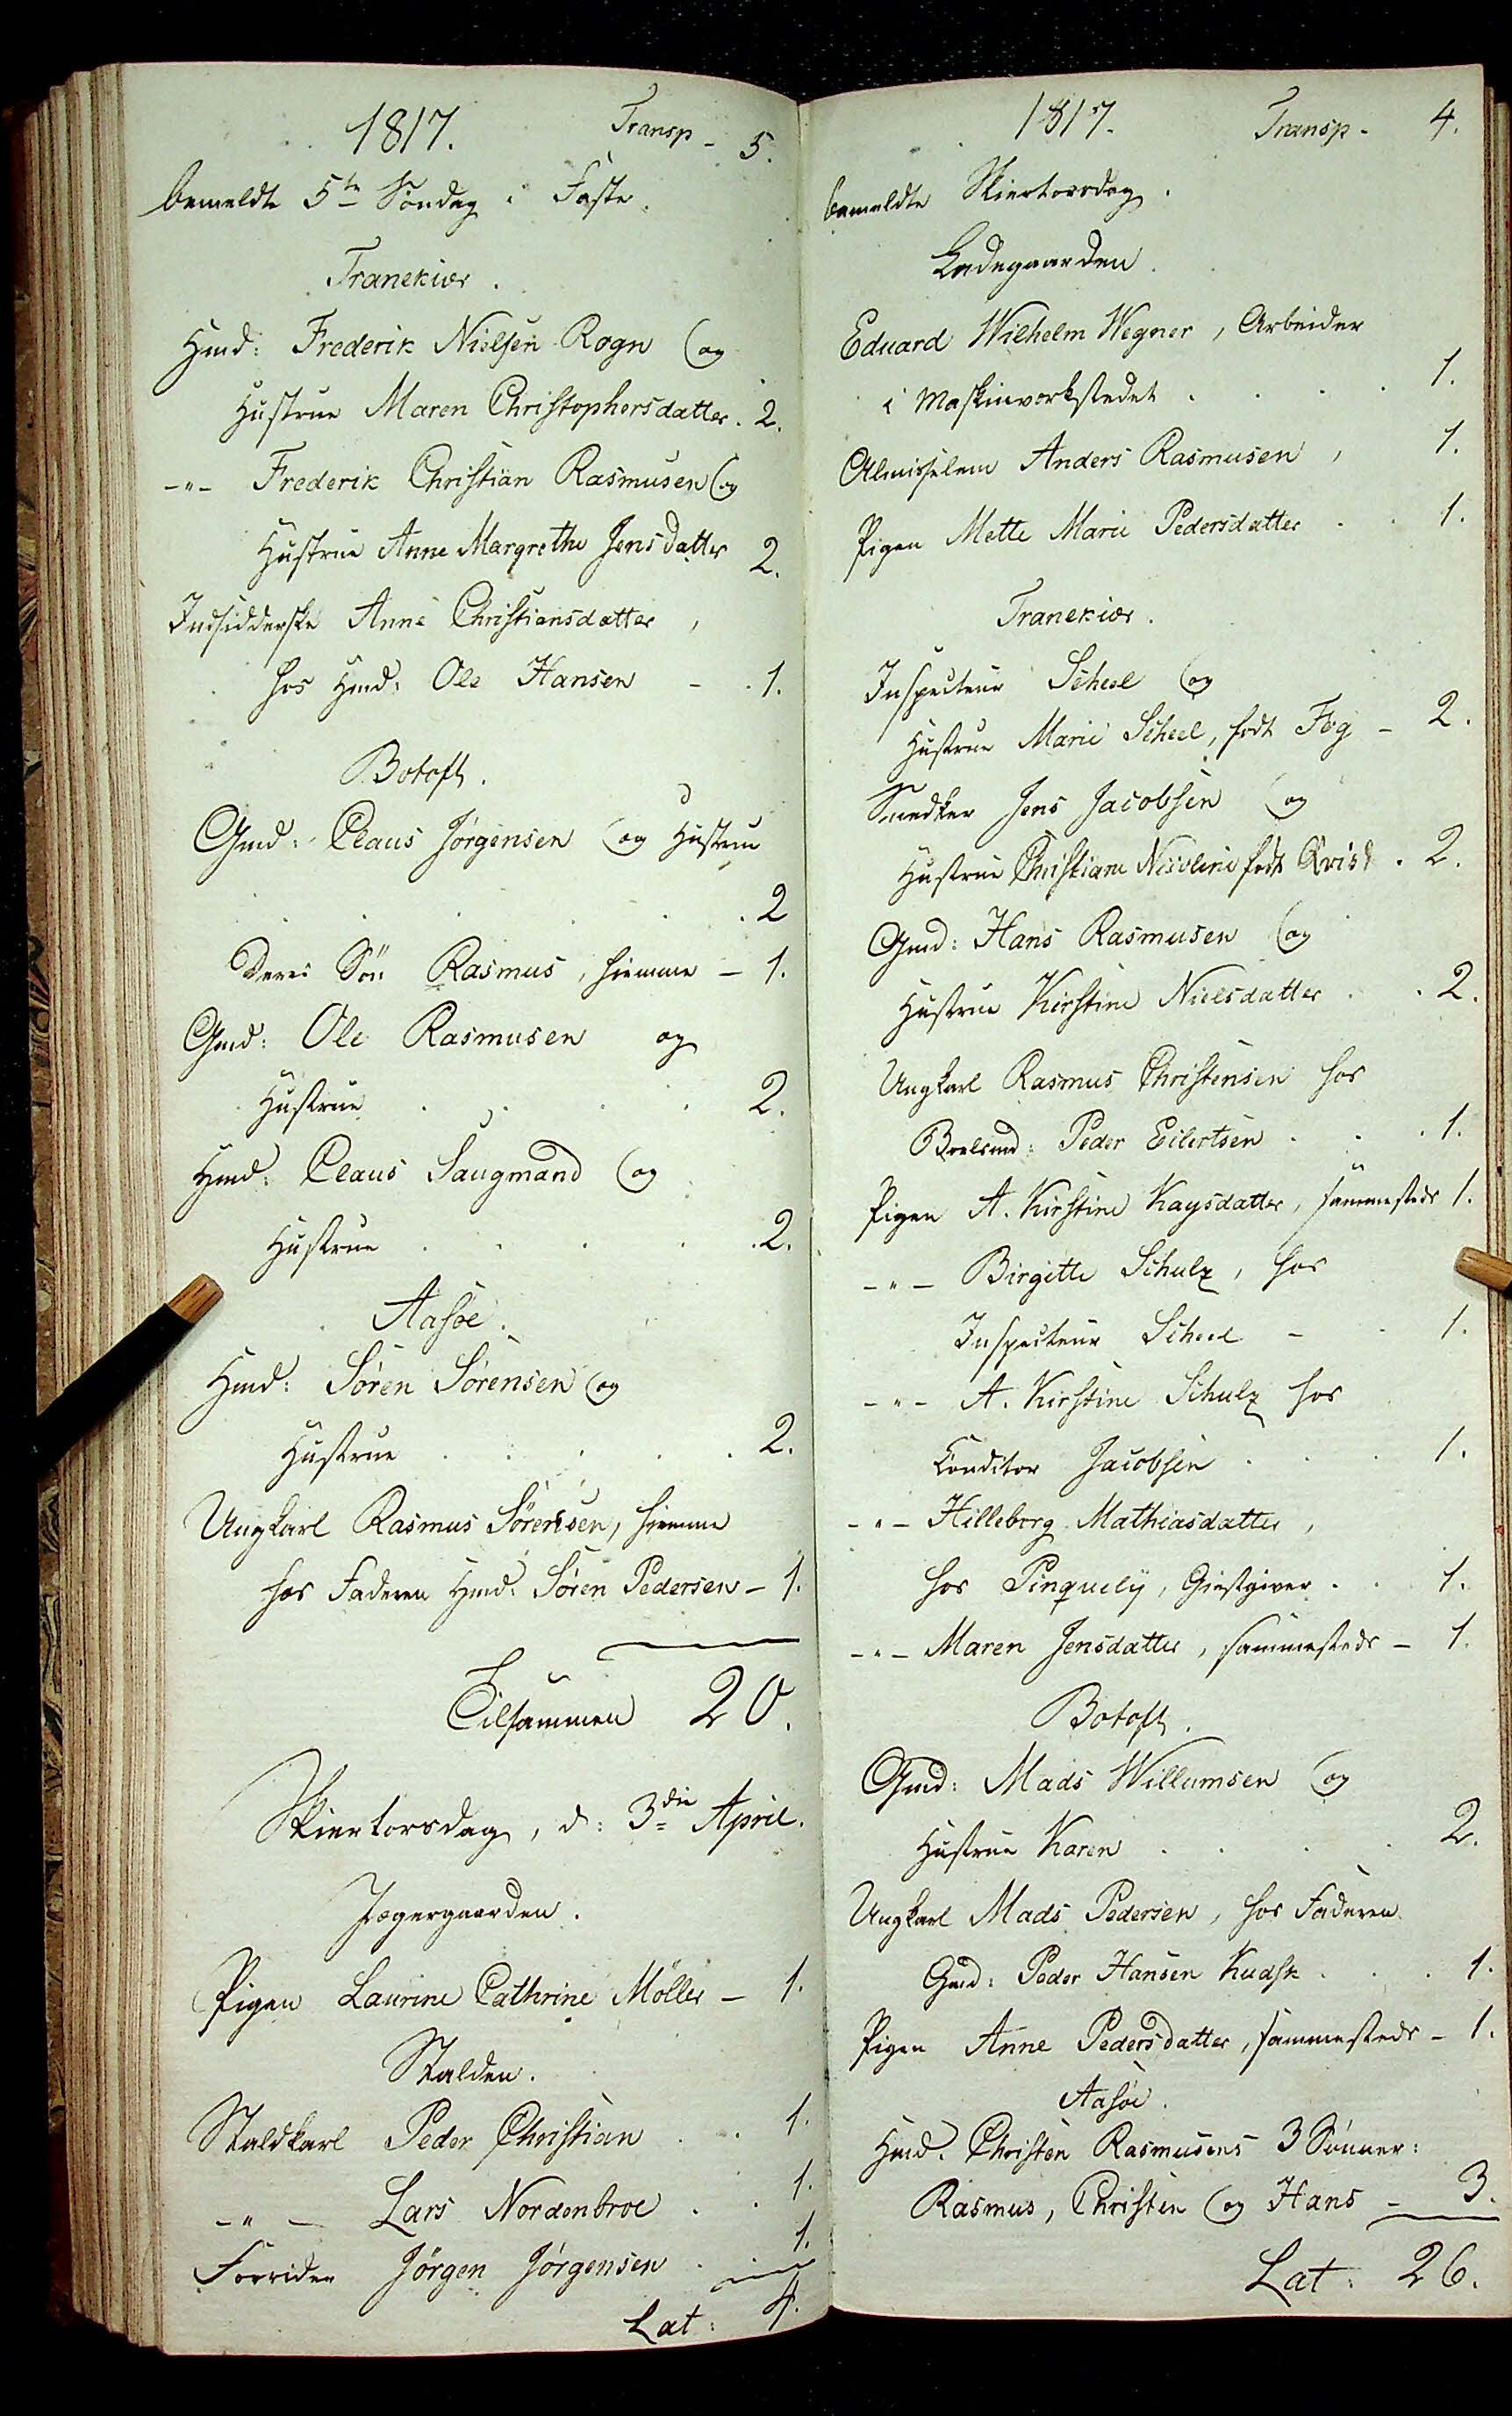1817.
Transp - 5.
bemeldte 5te Søndag i Faste
Tranekiær
Hmd: Frederik Nielsen Rogn og
Hustrue Maren Christophersdatter . 2.
-"- Frederik Christian Rasmusen og
Hustrue Anna Margrete JensDatter 2.
Indsidderske Anne Christiansdatter
hos Hmd: Ole Hansen - . 1.
Botoft.
Gmd: Claus Jørgensøn og Hustrue
. 2
Deres Søn Rasmus, hiemme - 1.
Gmd: Ole Rasmusen og
Hustrue . . . . 2.
Hmd Claus Saugmand og
Hustrue . . . . . 2.
Aasøe
Hmd: Søren Sørensen og
Hustrue . . . . . 2.
Ungkarl Rasmus Sørensen, hiemme
hos Faderen Hmd: Søren Pedersen - 1.
Tilsammen 20.
Skiertorsdag, d. 3die April.
Jægergaarden.
Pigen Laurine Cathrine Møller - 1.
Stalden.
Staldkarl Peder Christian . .1.
-"- Lars Nordenbroe . . 1.
Forider Jørgen Jørgensen . . 1.
Lat: 4
1817.
Transp . 4.
bemeldte Skiertorsdag
Ladegaarden.
Eduard Wilhelm Wegner, Arbeider
i Maskinvorkstedet . . . . 1.
Almisselem Anders Rasmusen, 1.
Pigen Mette Marie Pedersdatter . . 1.
Tranekiær.
Inspecteur Scheel og
Hustrue Marie Scheel, født Fog - 2.
Snedker Jens Jacobsen og
Hustrue Christiane Nicoline født Qvist . 2.
Gmd: Hans Rasmusen og
Hustrue Kirstine Nielsdatter . . 2.
Ungkarl Rasmus Christensen hos
Boelsmd: Peder Eilertsen . . . 1.
Pigen A. Kirstine Kaysdatter, sammesteds 1.
-"- Birgitte Schulz, hos
Inspecteur Scheel - . 1.
-"- A. Kirstine Schulz hos
Konditor Jacobsen . . . 1.
-"- Hilleborg Mathiasdatter
hos Pinquely, Gierstgiver . . 1.
-"- Maren Jensdatter, sammesteds - 1.
Botoft
Gmd: Mads Willumsen og
Hustrue Karen . . . . 2.
Ungkarl Mads Pedersen, hos Faderen
Gmd: Peder Hansen Kudsk . . . 1.
Pigen Anne Pedersdatter, sammesteds - 1
Aasøe
Hmd. Christen Rasmusens 3 Sønner:
Rasmus, Christen og Hans - 3
Lat: 26.
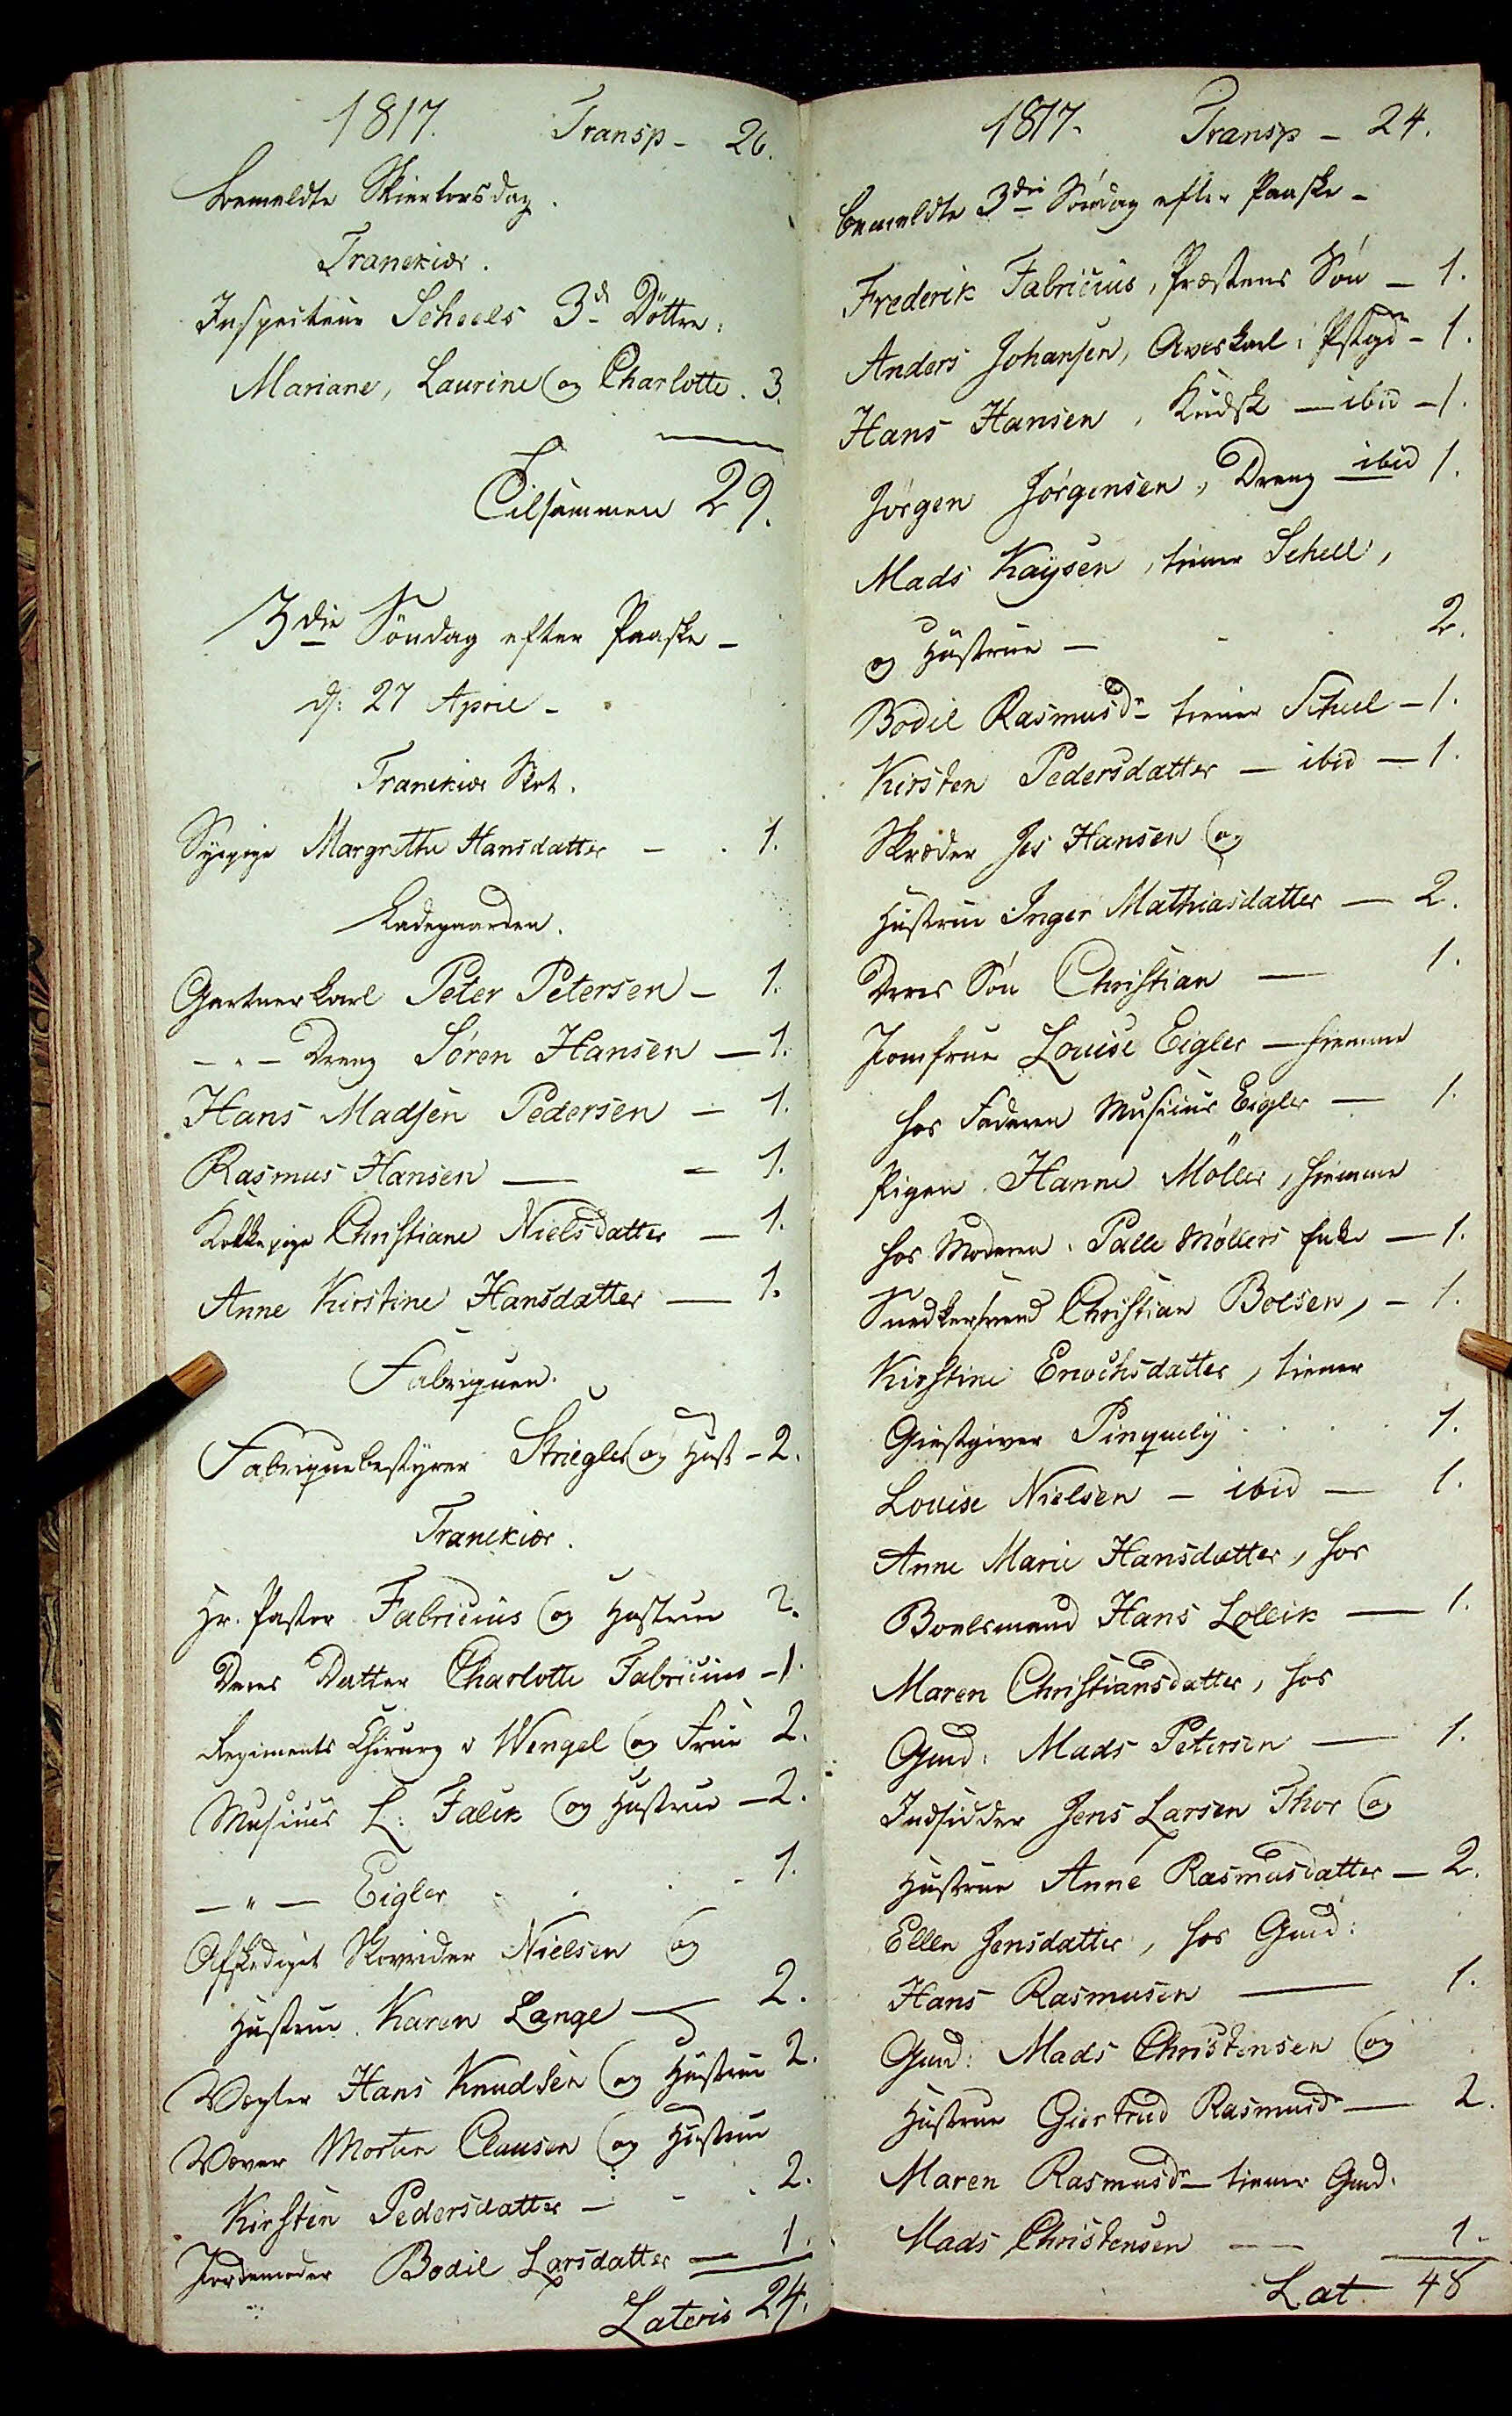1817.
Transp - 26.
bemeldte Skiertorsdag
Tranekiær
Inspecteur Scheels 3d Døttre:
Mariane, Laurine og Charlotte . 3.
Tilsammen 29.
3die Søndag efter Paaske-
d: 27 April-
Traneliær Slot.
Syepige Margrethe Hansdatter - . 1.
Ladegaarden.
Gartnerkarl Peder Petersen - 1.
-"- Dreng Søren Hansen - 1.
Hans Madsen Pedersen - 1.
Rasmus Hansen - - 1.
Kokkepige Christiane Nielsdatter - 1.
Anne Kirstine Hansdatter - 1.
Fabriquen.
Fabriquebestyrer Striegler og Hust - 2.
Tranekiær.
Hr. Pastor Fabricius og Hustrue 2.
Deres Datter Charlotte Fabricius - 1.
Regiments Chirurg v Wengel og Frue 2.
Musicus L: Falck og Hustrue - 2.
-"- Eigler . . . . 1.
Afskediget Skovrider Nielsen og
Hustrue Karen Lange - 2.
Vægter Hans Knudsen og Hustrue 2
Væver Morten Clausen og Hustrue
Kirstin Pedersdatter - - . 2.
Jordemoder Bodill Larsdatter - 1.
Lateris 24.
1817.
Transp - 24.
bemeldte 3die Søndag efter Paaske-
Frederik Fabricius, Præstens Søn - 1.
Anders Johansen, Avlskarl i Pstgd - 1
Hans Hansen, Kudsk - ibid - 1.
Jørgen Jørgensen, Dreng ibid - 1.
Mads Kaysen, tiener Scheel,
og Hustrue - . . 2.
Bodil Rasmusdr tiener Scheel - 1.
Kirsten Pedersdatter - ibid - 1.
Skræder Jes Hansen og
Hustrue Inger Mathiasdatter - 2.
Deres Søn Christian - 1.
Jomfrue Louise Eigler - hiemme
hos Faderen Musicus Eigler - 1.
Pigen Hans Møller, hiemme
hos Moderen: Palle Møllers Enke - 1.
Snedkersvend Christian Boesen, - 1.
Kirstine Enochsdatter, tiener
Giestgiver Pinquely . . . .1.
Louise Nielsen - ibid - 1.
Anne Marie Hansdatter, hos
Boelsmand Hans Lollik - 1.
Maren Christiansdatter, hos
Gmd: Mads Petersen - 1.
Indsidder Jens Larsen Thor og
Hustrue Anne Rasmusdatter - 2.
Ellen Jensdatter, hos Gmd:
Hans Rasmusen - 1.
Gmd: Mads Christensen og
Hustrue Giertrud Rasmusdr - 2.
Maren Rasmusdr tiener Gmd.
Madss Christensen - - 1.
Lat 48